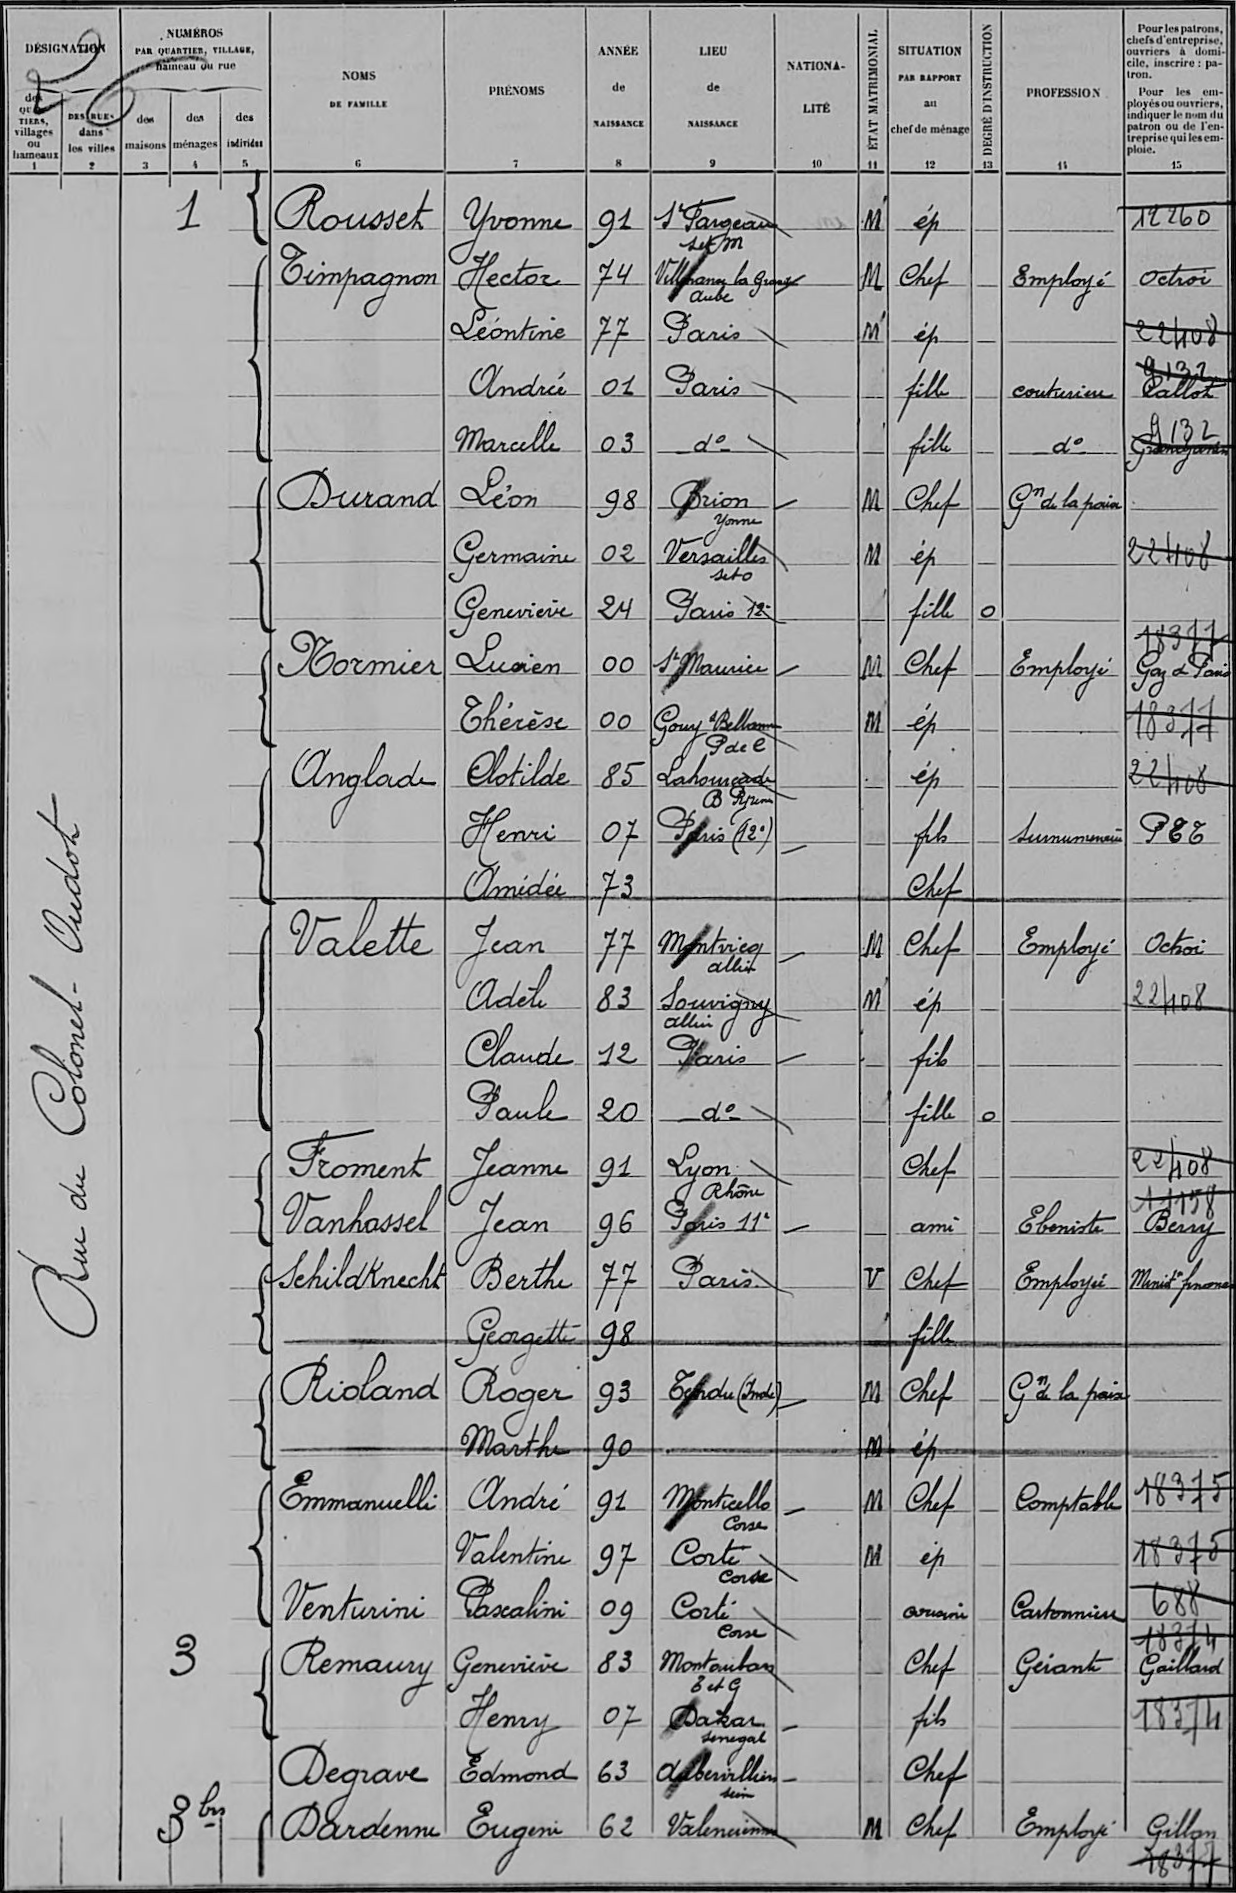Rousset/Yvonne/91/St Fargeau!s et m/¤/M/ép/¤/¤/12260
Timpagnon/Hector/74/Villenauxe la Grande! aube/¤/M/Chef/¤/Employé/octroi
¤/Léontine/77/Paris/¤/M/ép/¤/¤/22408
¤/Andrée/01/Paris/¤/¤/fille/¤/couturiere/Pallot?9132
¤/Marcelle/03/d°/¤/¤/fille/¤/d°/Grenguant ?9132
Durand/Léon/98/Brion ! Yonne/¤/M/Chef/¤/Gn de la paix/¤
¤/Germaine/02/Versailles!seto/¤/M/ép/¤/¤/22408
¤/Geneviève/24/Paris 12e/¤/¤/fille/o/¤/¤
Hormier/Lucien/00/St Maurice/¤/M/Chef/¤/Employé/Gaz de Paris?18377
¤/Thérèse/00/Gouy s Bellonne!P de C/¤/M/ép/¤/¤/18377
Anglade/Clotilde/85/Lahourcade!B Pyr/¤/¤/ép/¤/¤/22408
¤/Henri/07/Paris (12e)/¤/¤/fils/¤/surnuméraire/PTT
¤/Amédée/73/¤/¤/¤/Chef/¤/¤/¤
Valette/Jean/77/Montvicq!allier/¤/M/Chef/¤/Employé/Octroi
¤/Adèle/83/Souvigny!allier/¤/M/ép/¤/¤/22408
¤/Claude/12/Paris/¤/¤/fils/¤/¤/¤
¤/Paule/20/d°/¤/¤/fille/o/¤/¤
Froment/Jeanne/91/Lyon!Rhone/¤/¤/Chef/¤/¤/22408
Vanhassel/Jean/96/Paris 11e/¤/¤/ami/¤/Ebeniste/Berry ?11158
Sehildknecht/Berthe/77/Paris/¤/V/Chef/¤/Employée/Minist finances
¤/Georgette/98/¤/¤/¤/fille/¤/¤/¤
Rioland/Roger/93/Tendu (Indre)/¤/M/Chef/¤/Gn de la paix/¤
¤/Marthe/90/¤/¤/M/ép/¤/¤/¤
Emmanuelli/André/91/Monticello!Corse/¤/M/Chef/¤/Comptable/18375
¤/Valentine/97/Corte!Corse/¤/M/ép/¤/¤/18375
Venturini/Pascalini/09/Corte ! Corse/¤/¤/cousine/¤/Cartonnière/688
Remaury/Geneviève/83/Montauban!T et G/¤/¤/Chef/¤/Gérante/Gaillard?18374
¤/Henry/07/Dakar!Senegal/¤/¤/fils/¤/¤/18374
Degrave/Edmond/63/Aubervilliers!Seine/¤/¤/Chef/¤/¤/¤
Dardenne/Eugenie/62/Valenciennes/¤/M/Chef/¤/Employé/Gillon!18377
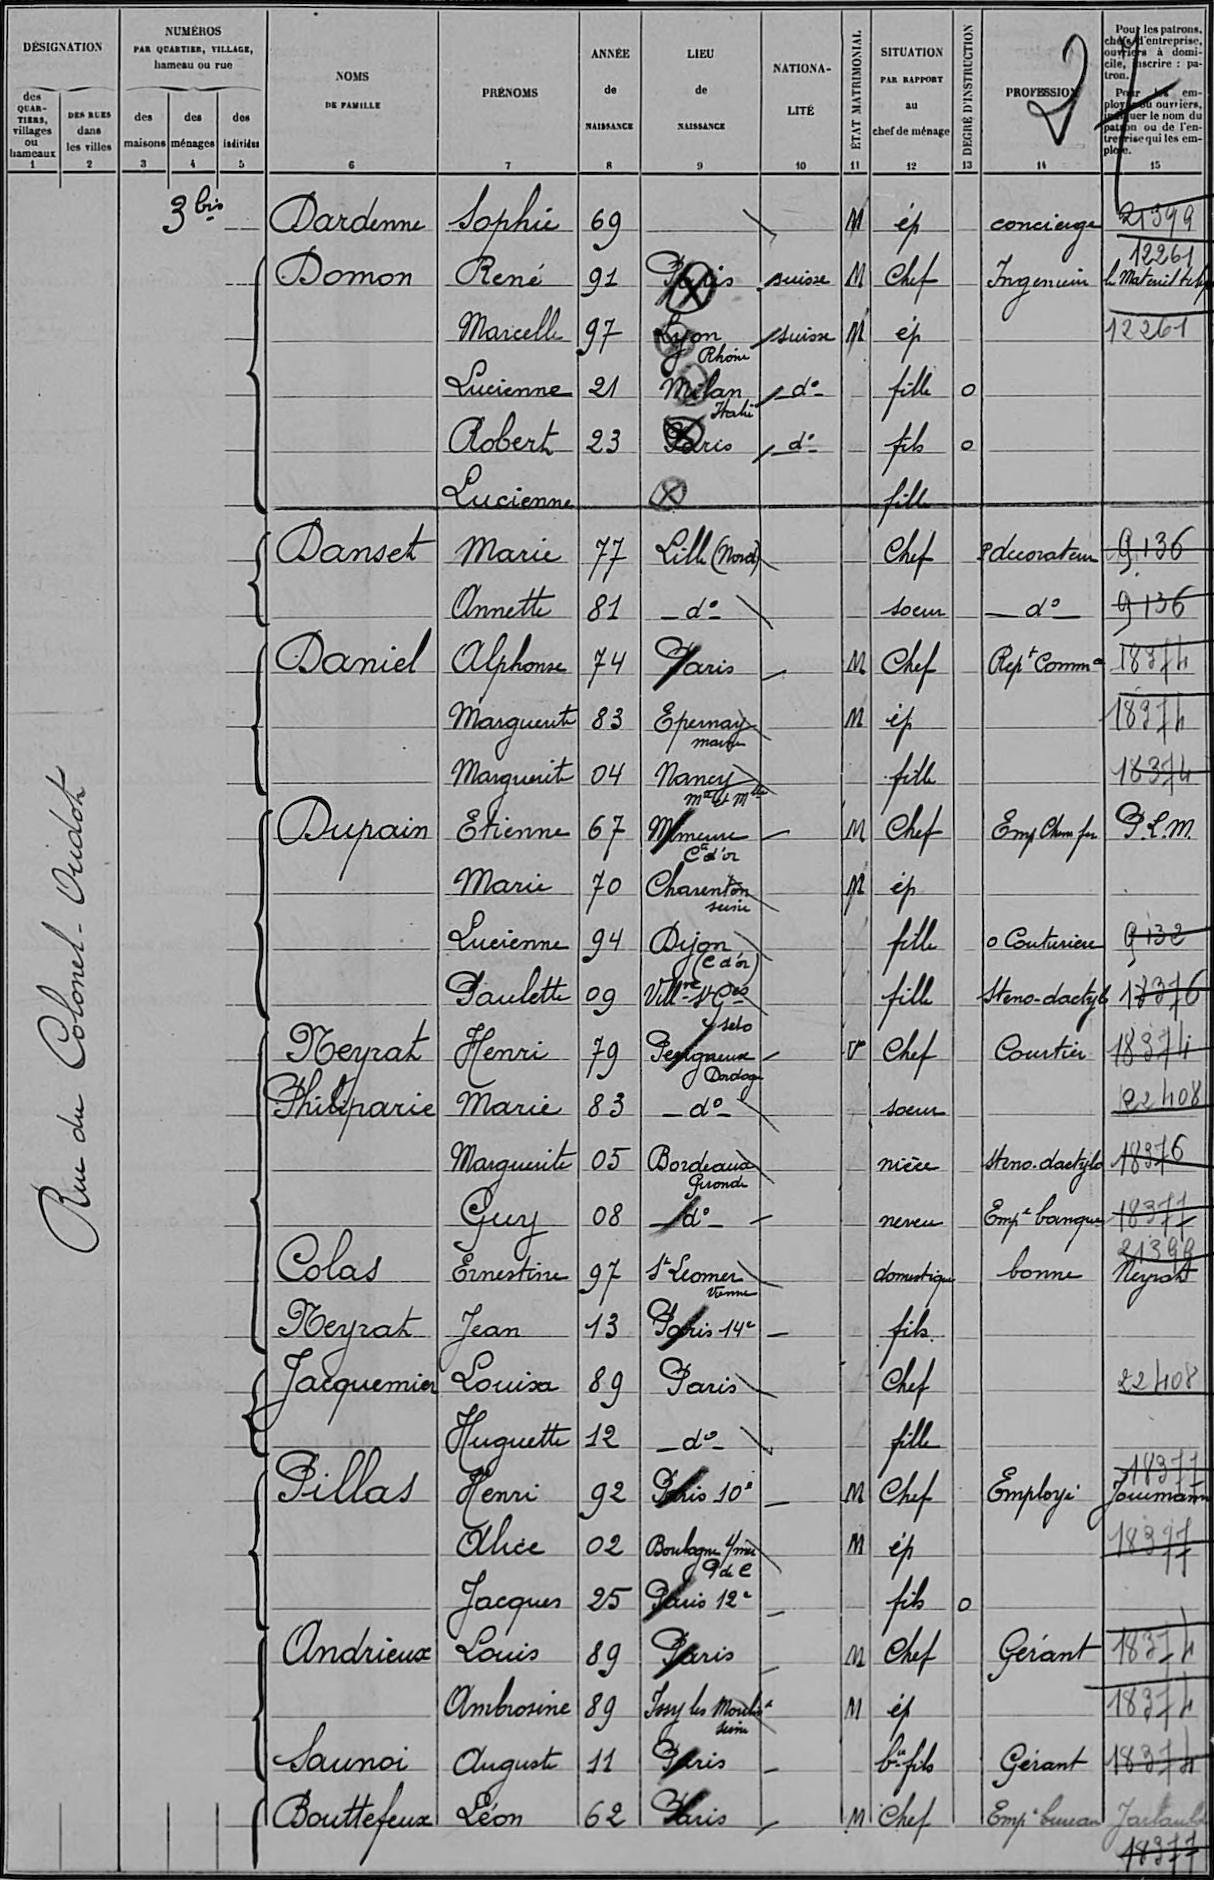Dardenne/Sophie/69/¤/¤/M/ép /¤/concierge/21399
Domon/René/91/Paris/Suisse/M/chef/¤/Ingenieur/l materiel tchq?12261
¤/Marcelle/97/Lyon!Rhône/Suisse/M/ép/¤/¤/12261
¤/Lucienne/21/Milan ! Italie/d°/¤/fille/o/¤/¤
¤/Robert/23/Paris/d°/¤/fils/o/¤/¤
¤/Lucienne/¤/¤/¤/¤/fille/¤/¤/¤
Danset/Marie/77/Lille (Nord)/¤/¤/Chef/¤/P decorateur/9136
¤/Annette/81/d°/¤/¤/soeur/¤/d°/9136
Daniel/Alphonse/74/Paris/¤/M/Chef/¤/Rept Comm/18374
¤/Marguerite/83/Epernay!marne/¤/M/ép/¤/¤/18374
¤/Marguerite/04/Nancy!mte et mlle/¤/¤/fille/¤/¤/18374
Dupain/Etienne/67/Mimeure!Cte d'or/¤/M/Chef/¤/Emp chm fer/P L M
¤/Marie/70/Charenton ! seine/¤/M/ép/¤/¤/¤
¤/Lucienne/94/Dijon!(C d'or)/¤/¤/fille/¤/o Couturiere/9132
¤/Paulette/09/Vill-St-Ges!seto/¤/¤/fille/¤/steno-dactylo/18376
Merpat/Henri/79/Perigueux!Dordogne/¤/V/Chef/¤/Courtier/18374
Philiparie/Marie/83/d°/¤/¤/soeur/¤/¤/22408
¤/Marguerite/05/Bordeaux!Gironde/¤/¤/nièce/¤/steno dactylo/18376
¤/Guy/08/d°/¤/¤/neveu/¤/Emp banque/18377
Colas/Ernestine/97/St Leomer!Vienne/¤/¤/domestique/¤/bonne/Neyrant?21399
Meyrat/Jean/13/Paris 14e/¤/¤/fils/¤/¤/¤
Jacquemier/Louisa/89/Paris/¤/¤/Chef/¤/¤/22408
¤/Huguette/12/d°/¤/¤/fille/¤/¤/¤
Pillas/Henri/92/Paris 10e/¤/M/Chef/¤/Employé/Jourmann?18377
¤/Alice/02/Boulogne s: mer!P de C/¤/M/ép/¤/¤/18377
¤/Jacques/25/Paris 12e/¤/¤/fils/o/¤/¤
Andrieux/Louis/89/Paris/¤/M/Chef/¤/Gérant/18374
¤/Ambrosine/89/Issy les Mouli!seine/¤/M/ép/¤/¤/18374
Saunoi/Auguste/11/Paris/¤/¤/b Fils/¤/Gérant/18374
Bouttefeux/Léon/62/Paris/¤/M/Chef/¤/Emp bureau/Jartaud!18377